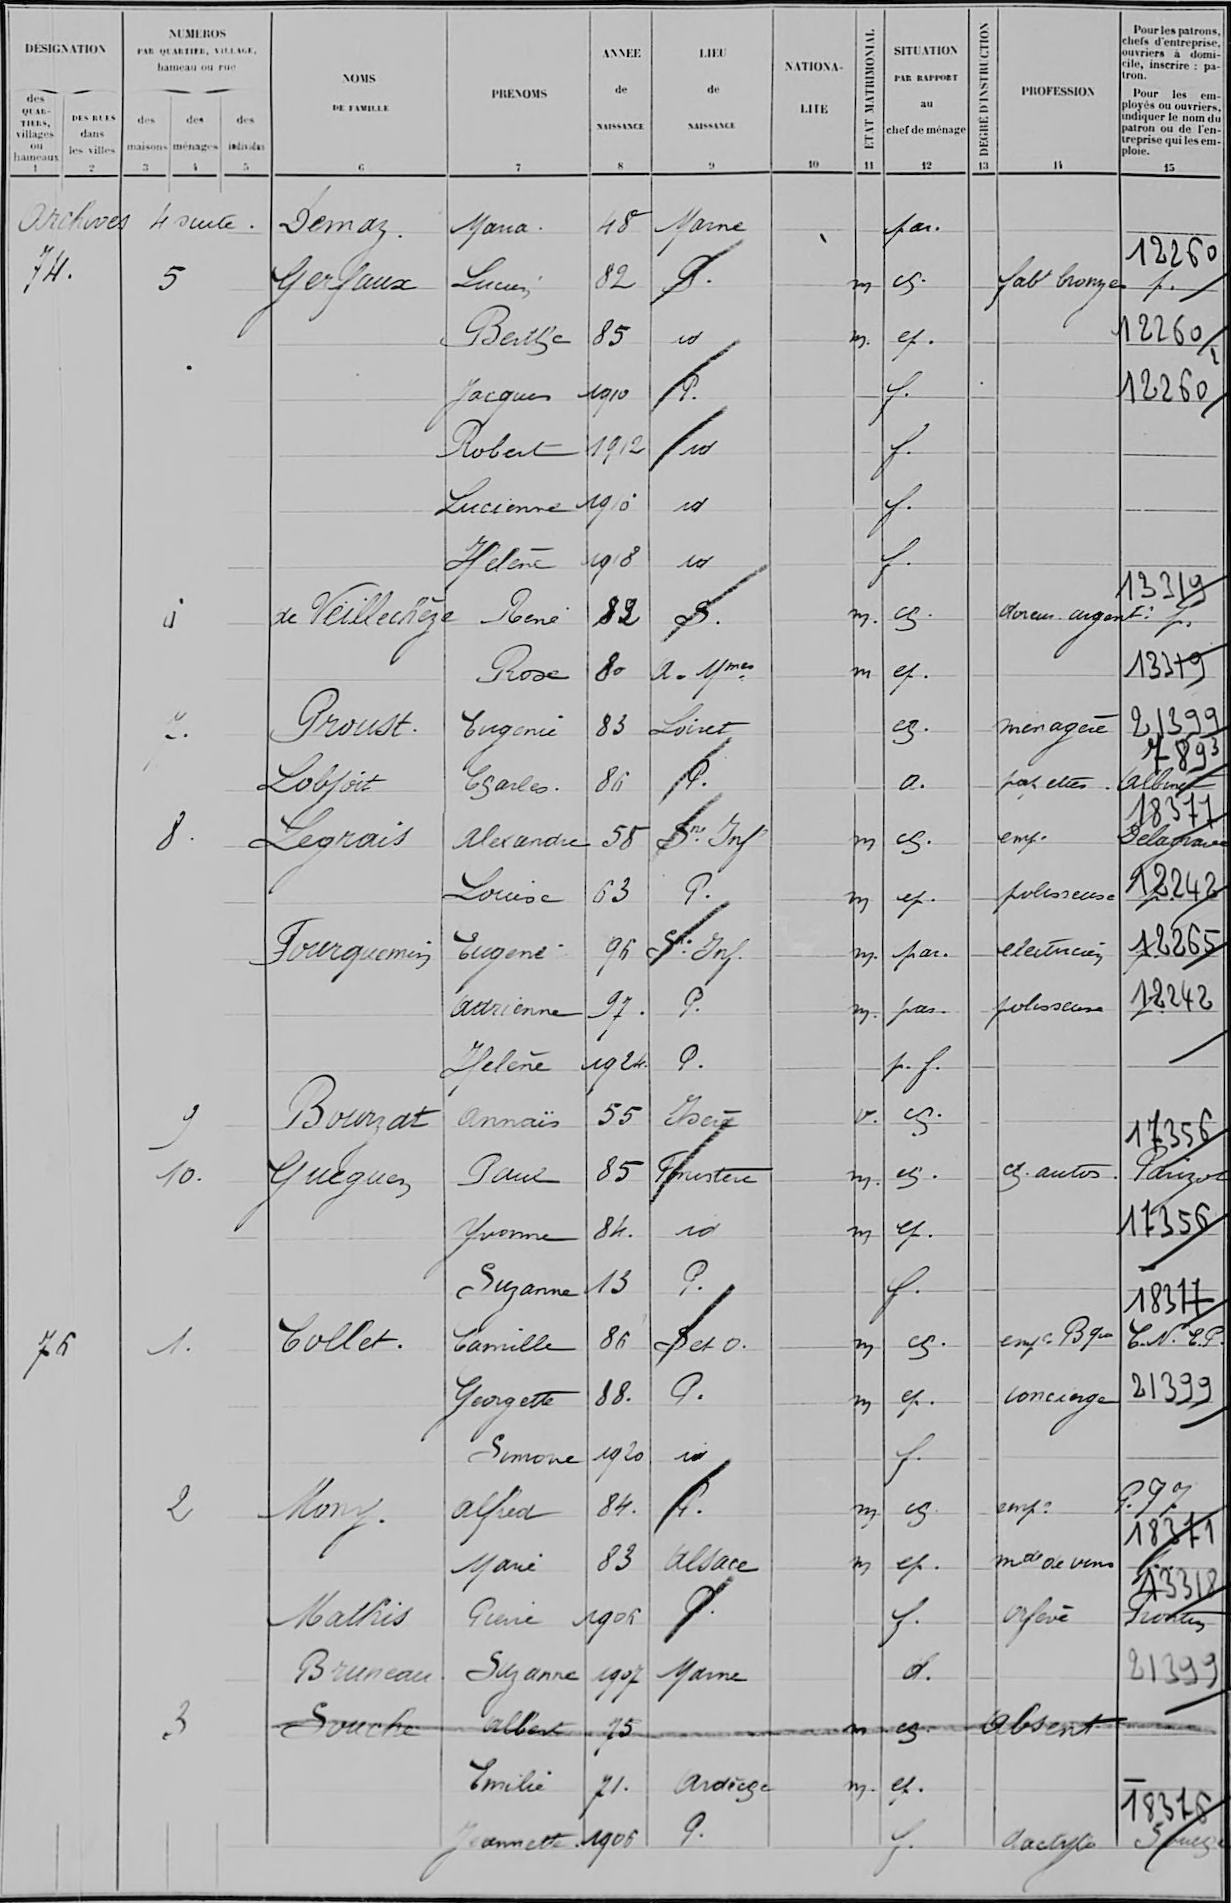Demaz/Maria/48/Marne/¤/¤/par /¤/¤/¤
Gerfaux/Lucien/82/P /¤/m /cf /¤/fabt bronze p /?12260
¤/Berthe/85/id/¤/m /ep /¤/¤/12260
¤/Jacques/1910/P /¤/¤/f /¤/¤/12260
¤/Robert/1912/id/¤/¤/f /¤/¤/¤
¤/Lucienne/1910/id/¤/¤/f /¤/¤/¤
¤/Helène/1918/id/¤/¤/f /¤/¤/¤
de Veillechèze/René/82/S /¤/m /cf /¤/doreur argent f /?13319
¤/Rose/80/A Mmes/¤/m/ep /¤/¤/13319
Proust/Eugénie/83/Loiret/¤/¤/ep /¤/ménagère/21399
Lobjoit/Charles/86/P /¤/¤/a /¤/panetier /Albinet?789
Legrais/alexandre/58/sne Inf/¤/m/cf /¤/emp /Delagraive?18377
¤/Louise/63/P /¤/m/ep /¤/polisseuse/12242
Fourquemin/Eugène/96/Sne Inf /¤/m /par /¤/electricien/12265
¤/Adrienne/97/P /¤/m /par /¤/polisseuse/12242
¤/Hélène/1924/P /¤/¤/p f /¤/¤/¤
Bourzat/Annaïs/55/Isère/¤/v /cf /¤/¤/¤
Gueguen/Paul/85/Finistère/¤/m /ef /¤/cf autos /Parizot!17356
¤/Yvonne/84/id/¤/m/ep /¤/¤/17356
¤/Suzanne/13/P /¤/¤/f /¤/¤/¤
Collet/Camille/86/S et O /¤/m/cf /¤/empe Bque/C N E P ?18377
¤/Georgette/88/P /¤/m/ep /¤/concierge/21399
¤/Simone/1920/id/¤/¤/f /¤/¤/¤
Mony/Alfred/84/P /¤/m/cf /¤/emp /P T T
¤/Marie/83/Alsace/¤/m/ep /¤/mde de vins/?18371
Mathis/Pierre/1906/P /¤/¤/f /¤/Orfèvre/Prontin?13318
Bruneau/Suzanne/1907/Marne/¤/¤/d /¤/¤/21399
Souche/Albert/75/¤/¤/m /ch/¤/absent/¤
¤/Emilie/71/Ardèche/¤/m /ep /¤/¤/¤
¤/Jeannette/1906/P /¤/¤/f /¤/dactylo/Souège?18376
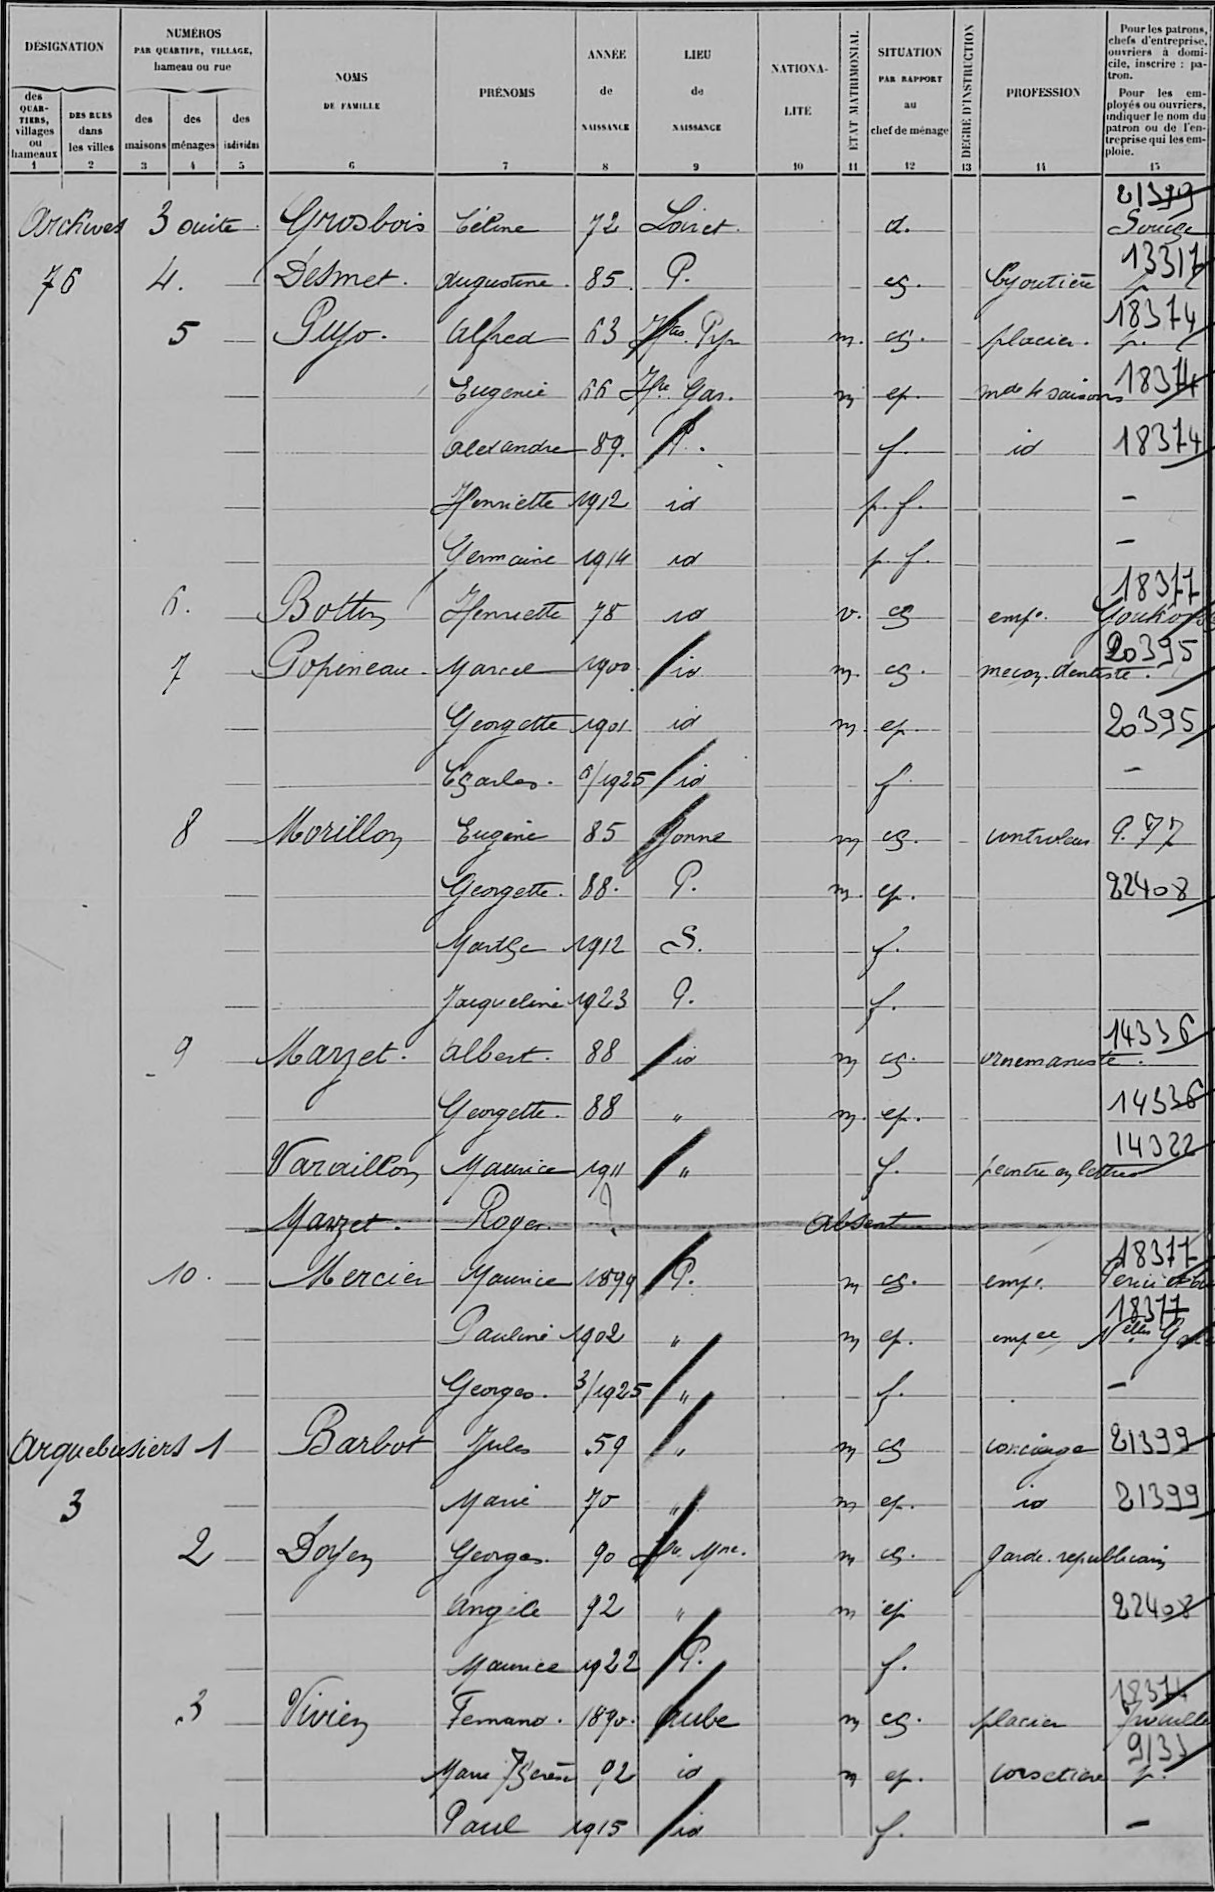Grosbois/Céline/72/Loiret/¤/¤/d /¤/¤/Soriège?21399
Desmet/Augustine/85/P /¤/¤/ch /¤/bijoutière/13317
Pujo/Alfred/63/Htes Pyr/¤/m /ch /¤/placier /p ?18374
¤/Eugénie/66/Hte Gar /¤/m/ep /¤/made 4 Saisons/18374
¤/Alexandre/89/P /¤/¤/f /¤/id/18374
¤/Henriette/1912/id/¤/¤/p f /¤/¤/¤
¤/Germaine/1914/id/¤/¤/p f /¤/¤/¤
Bottin/Henriette/78/id/¤/v /ch/¤/emp°/Goukor?18377
Popineau/Marcel/1900/id/¤/m /ch /¤/mecan dentiste/?20395
¤/Georgette/1901/id/¤/m /ep /¤/¤/20395
¤/Charles/6:1925/id/¤/¤/f /¤/¤/¤
Morillon/Eug-ne/85/Yonne/¤/m/ch /¤/controleur/P T T
¤/Georgette/88/P /¤/m /ep /¤/¤/22408
¤/Marthe/1912/S /¤/¤/f /¤/¤/¤
¤/Jacqueline/1923/P /¤/¤/f /¤/¤/¤
Marzet/Albert/88/id/¤/m/ch/¤/ornemaniste/?14336
¤/Georgette/88/¤/¤/m/ep /¤/¤/14336
Varaillon/Maurice/1911/¤/¤/¤/f /¤/peinte au lettres/?14322
Marzet/Roger/?/¤/absent/¤/¤/¤/¤/¤
Mercier/Maurice/1899/P /¤/m /ch /¤/emp /Perici et Cie ?18377
¤/Pauline/1902/"/¤/m/ep /¤/empee /Nelle Gal?18377
¤/Georges/3:1925/¤/¤/¤/f /¤/¤/¤
Barbot/Jules/59/¤/¤/m/ep /¤/concierge/21399
¤/Marie/70/¤/¤/m /ep /¤/io/21399
Doyen/georges/90/Hte Mne/¤/m/ch /¤/Garde republicain/¤
¤/Angèle/92/¤/¤/m/ep/¤/¤/22408
¤/Maurice/1922/P /¤/¤/f /¤/¤/¤
Vivien/Fernand/1890/Aube/¤/m/ch /¤/placier/Trouille?18374
¤/Marie Thérèse/92/id/¤/m/ep /¤/corsetière/p ?9133
¤/Paul/1915/id /¤/¤/f /¤/¤/¤